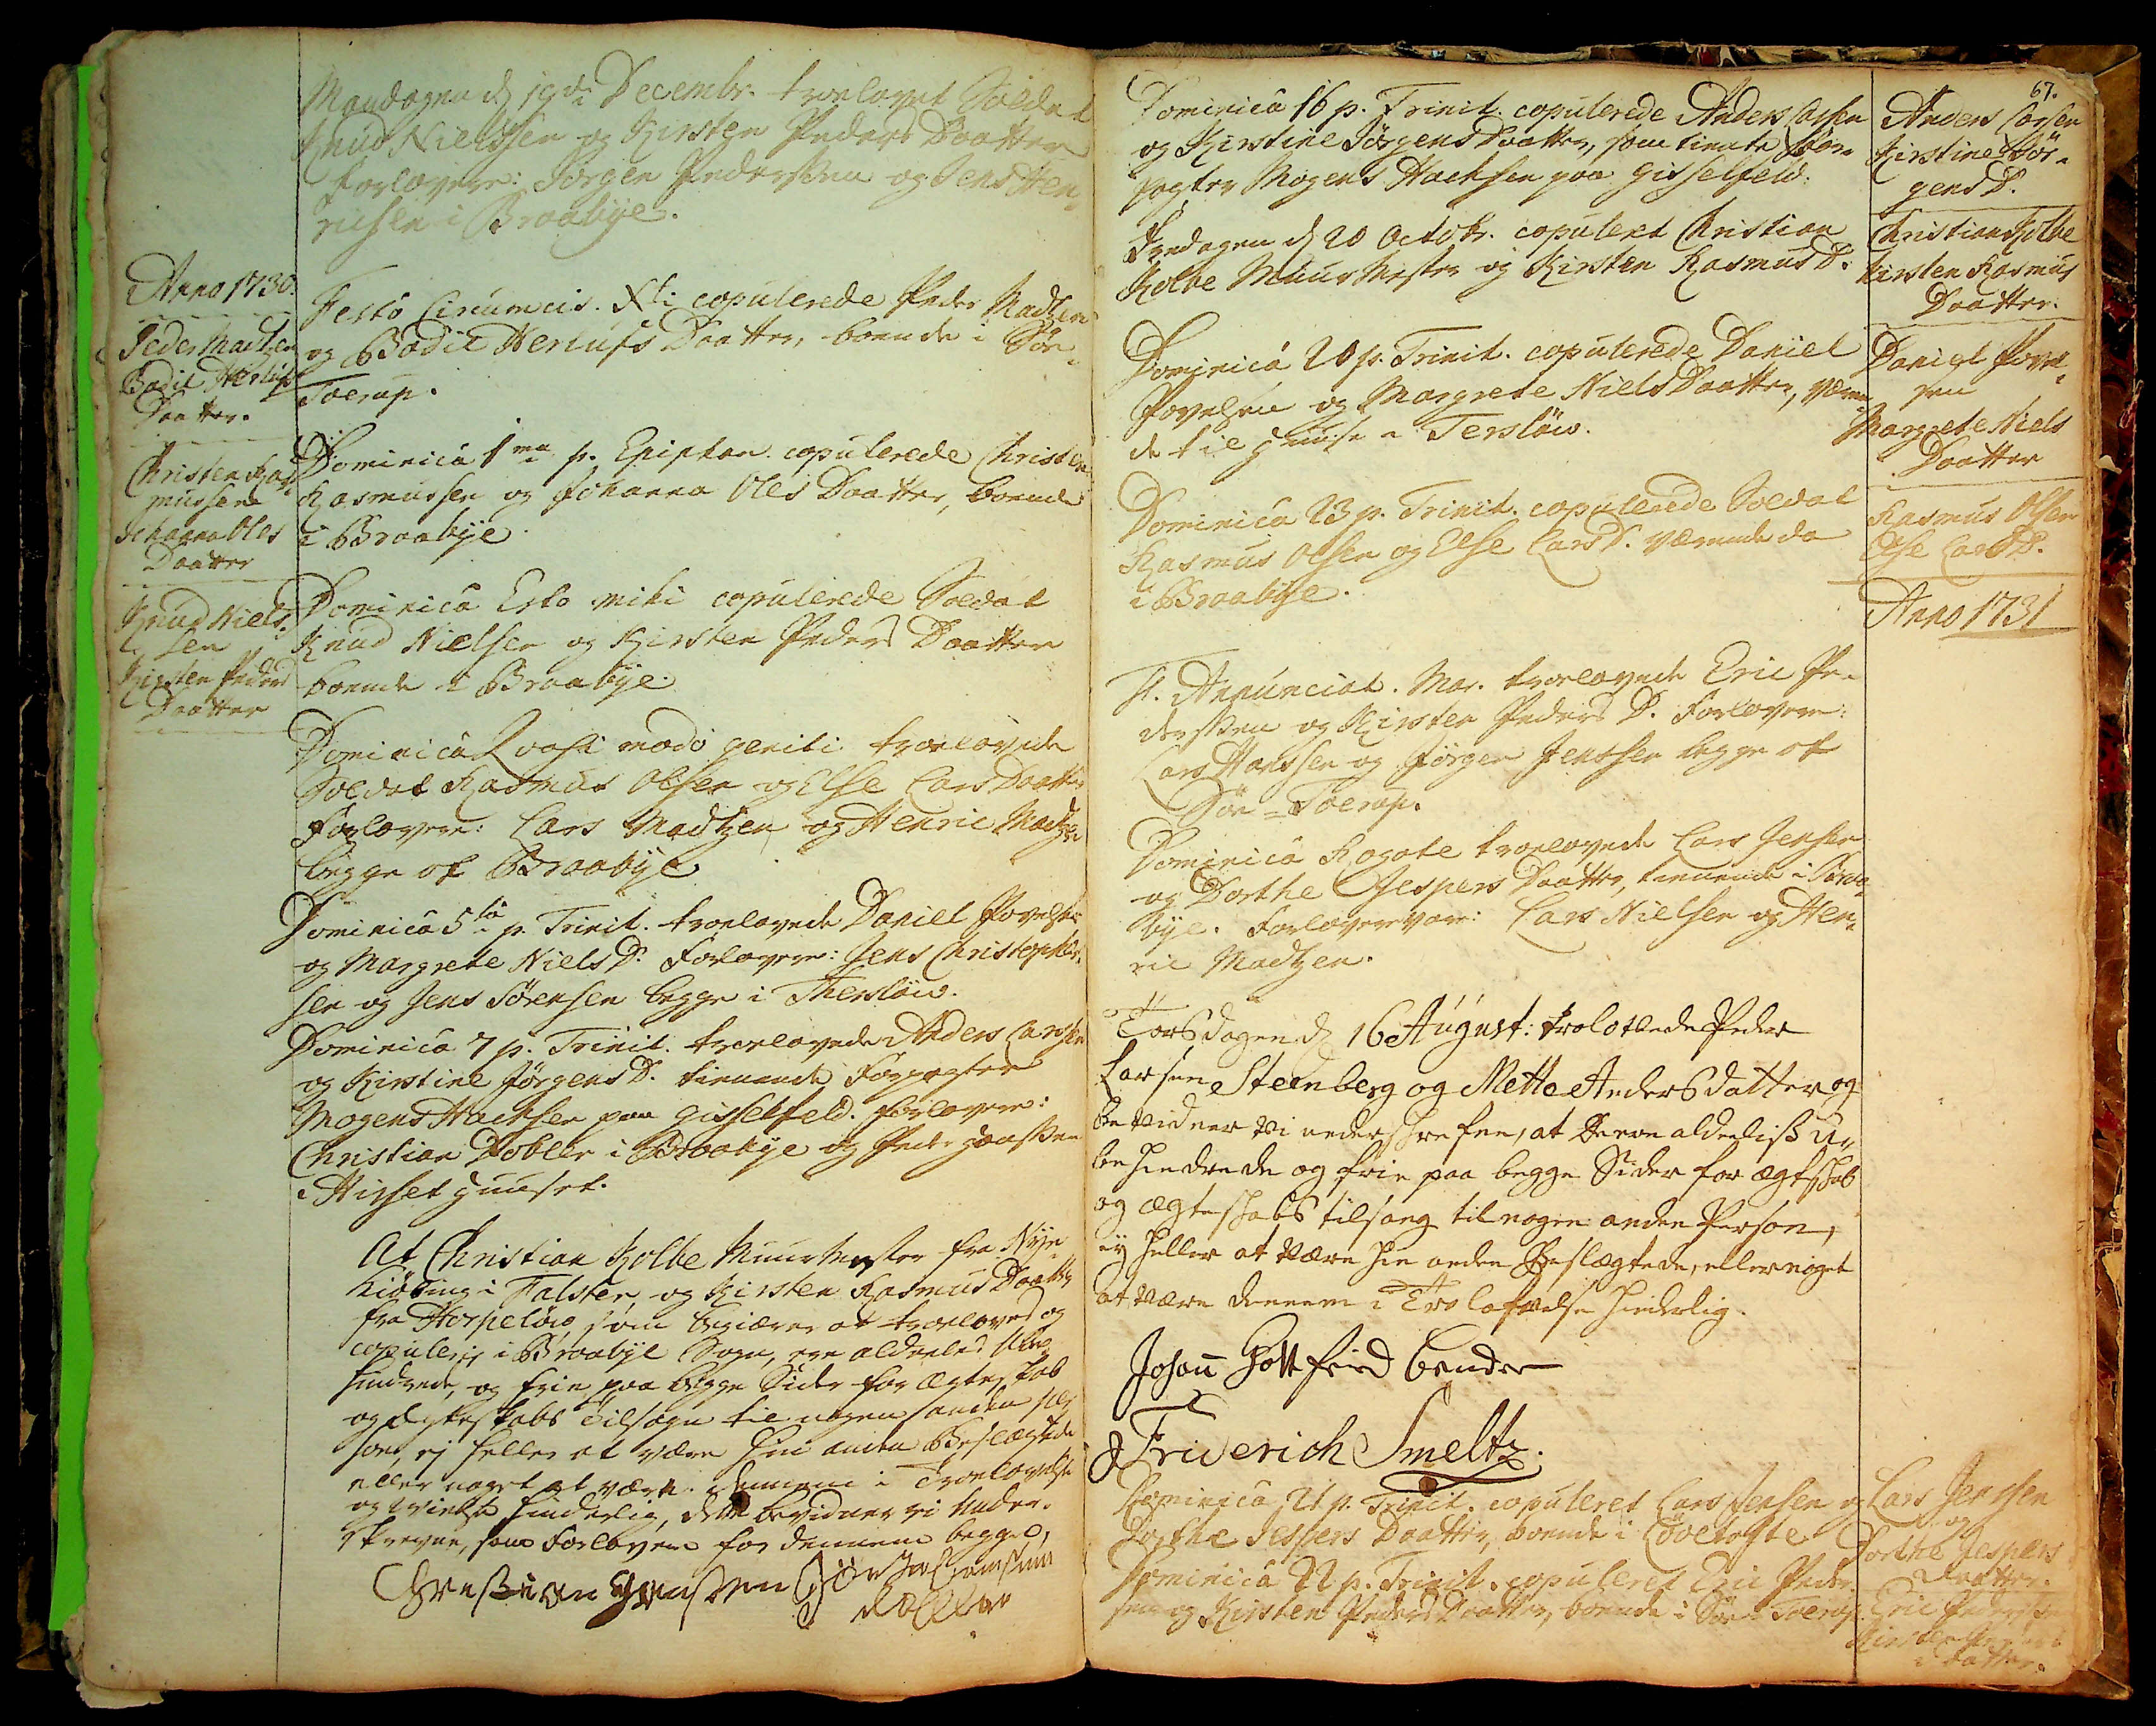Mandagen d. 19da## Decembr. troelovet Soldat
Knud Nielssen og Kirsten Peders Daatter.
Forlovere: Jørgen Pederssen og Jens Hen¬
ricsen i Braabye. 
Anno 1730
Peder Madtzen 
Bodil Herlufs
Daatter 
Festo Circumcis. Xte copulerede Peder Madtzen
og Bodil Herlufs Daatter, boende i Søe 
Toerup.
Christen Ras¬
mussen 
Johanna Oles
Daatter 
Dominica 1ma p. Epiphan. copulerede Christen
Rasmussen og Johanna Oles Daatter, boende
i Braabye.
Knud Niels¬
sen 
Kirsten Peders
Daatter
Dominica Esto Mihi copulerede Soldat
Knud Nielsen og Kirsten Peders Daatter
boende i Braabye. 
Dominica Quasimodo geniti troelovede
Soldat Rasmus Olsen og Else Lars Daatter.
Forlovere: Lars Madtzen og Henric Madtzen
begge af Braabye.
Dominica 5ta p. Trinit. troelovet Daniel Povelsen
og Margrete NielsD. Forlovere: Jens Christopher¬
sen og Jens Sørensen, begge i Frersløv.
Dominica 7 p. Trinit. troelovede Anders Larsen
og Kirstine JørgensD. tienende Forpagter
Mogens Hacksen## paa Gisselfeld. Forlovere:
Christian Dobler i Braabye og Peder Hanssen
i Hirset Huuset.
At Christian Kolbe MuurMester fra Nye¬
Kiøbing i Falster, og Kirsten Rasmus Daatter
fra Horpeløv, som begiærer at troeloves og
copuleris i Braabye Sogn, ere aldeeles uden
hindrer og frie paa begge sider for Ægteskab
og Ægteskabs Tilsagn til nogen anden per
son ej heller at være Hinanden Beslægtede
eller noget at være## dennem i Trolovelse
og Vielse hinderlig, dette bevidner vi under¬
skrevne som Forlovere for dennem begge.
Christian Iversen## Jørgen Hansen##
Dobler##
Dominica 16 p. Trinit. copulerede Anders Larsen
og Kirstine Jørgens Daatter, som tiente For
pagter Mogens Hacksen## paa Gisselfeld.
67.
Anders Larsen
Kirstine Jør¬
gensD.
Fredagen d. 20 Octobr. copuleret Christian
Kolbe MuurMester og Kirsten RasmusD.
Christian Kolbe
Kirsten Rasmus
Daatter 
Dominica 20 p.Trinit copulerede Daniel 
Povelsen og Margrete Niels Daatter, væren¬
de til Huuse i Tersløv.
Daniel Povel¬
sen 
Margrete Niels 
Daatter 
Dominica 23 p. Trinit copulerede Soldat 
Rasmus Olsen og Else LarsD. Værende da
i Braabye.
Rasmus Olesen 
Else LarsD.
F. Annunciat Mar. troelovet Eric Pe¬
derssen og Kirsten PedersD. Forlovere:
Lars Hanssen og Jørgen Jenssen begge af
Søe Toerup.
Anno 1731
Dominica Rogate troelovet Lars Jenssen
og Dorthe Jespers Daatter tienende i Braa##¬
bye. Forlovere vare: Lars Nielsen og Hen¬
ric Madtzen.
Torsdagen d. 16 August: troelovede Peder
Larsen Steenberg og Mette Andersdatter og
bevidner vi underskrefne at de ere aldelis u¬
den## hindrende og frie paa begge Sider for Ægteskab
og Ægteskabs tilsang til nogen anden Person,
ey heller at Være hinanden Beslægtede eller noget
at Være dennem i Trolofvelse hinderlig.
Johan Gottfred bonde
Frederich Smeltz 
Dominica 21 p. Trinit. copuleret Lars Jensen og
Dorthe Jespers Daatter, boende i Løvetofte.
Lars Jensen 
og
Dorthe Jespers
Daatter.
Dominica 22 p. Trinit. copuleret Eric Peder¬
sen og Kirsten Peders Daatter, boende i Søe-Toerup.
Eric Pedersen
Kirsten Peders
Datter.
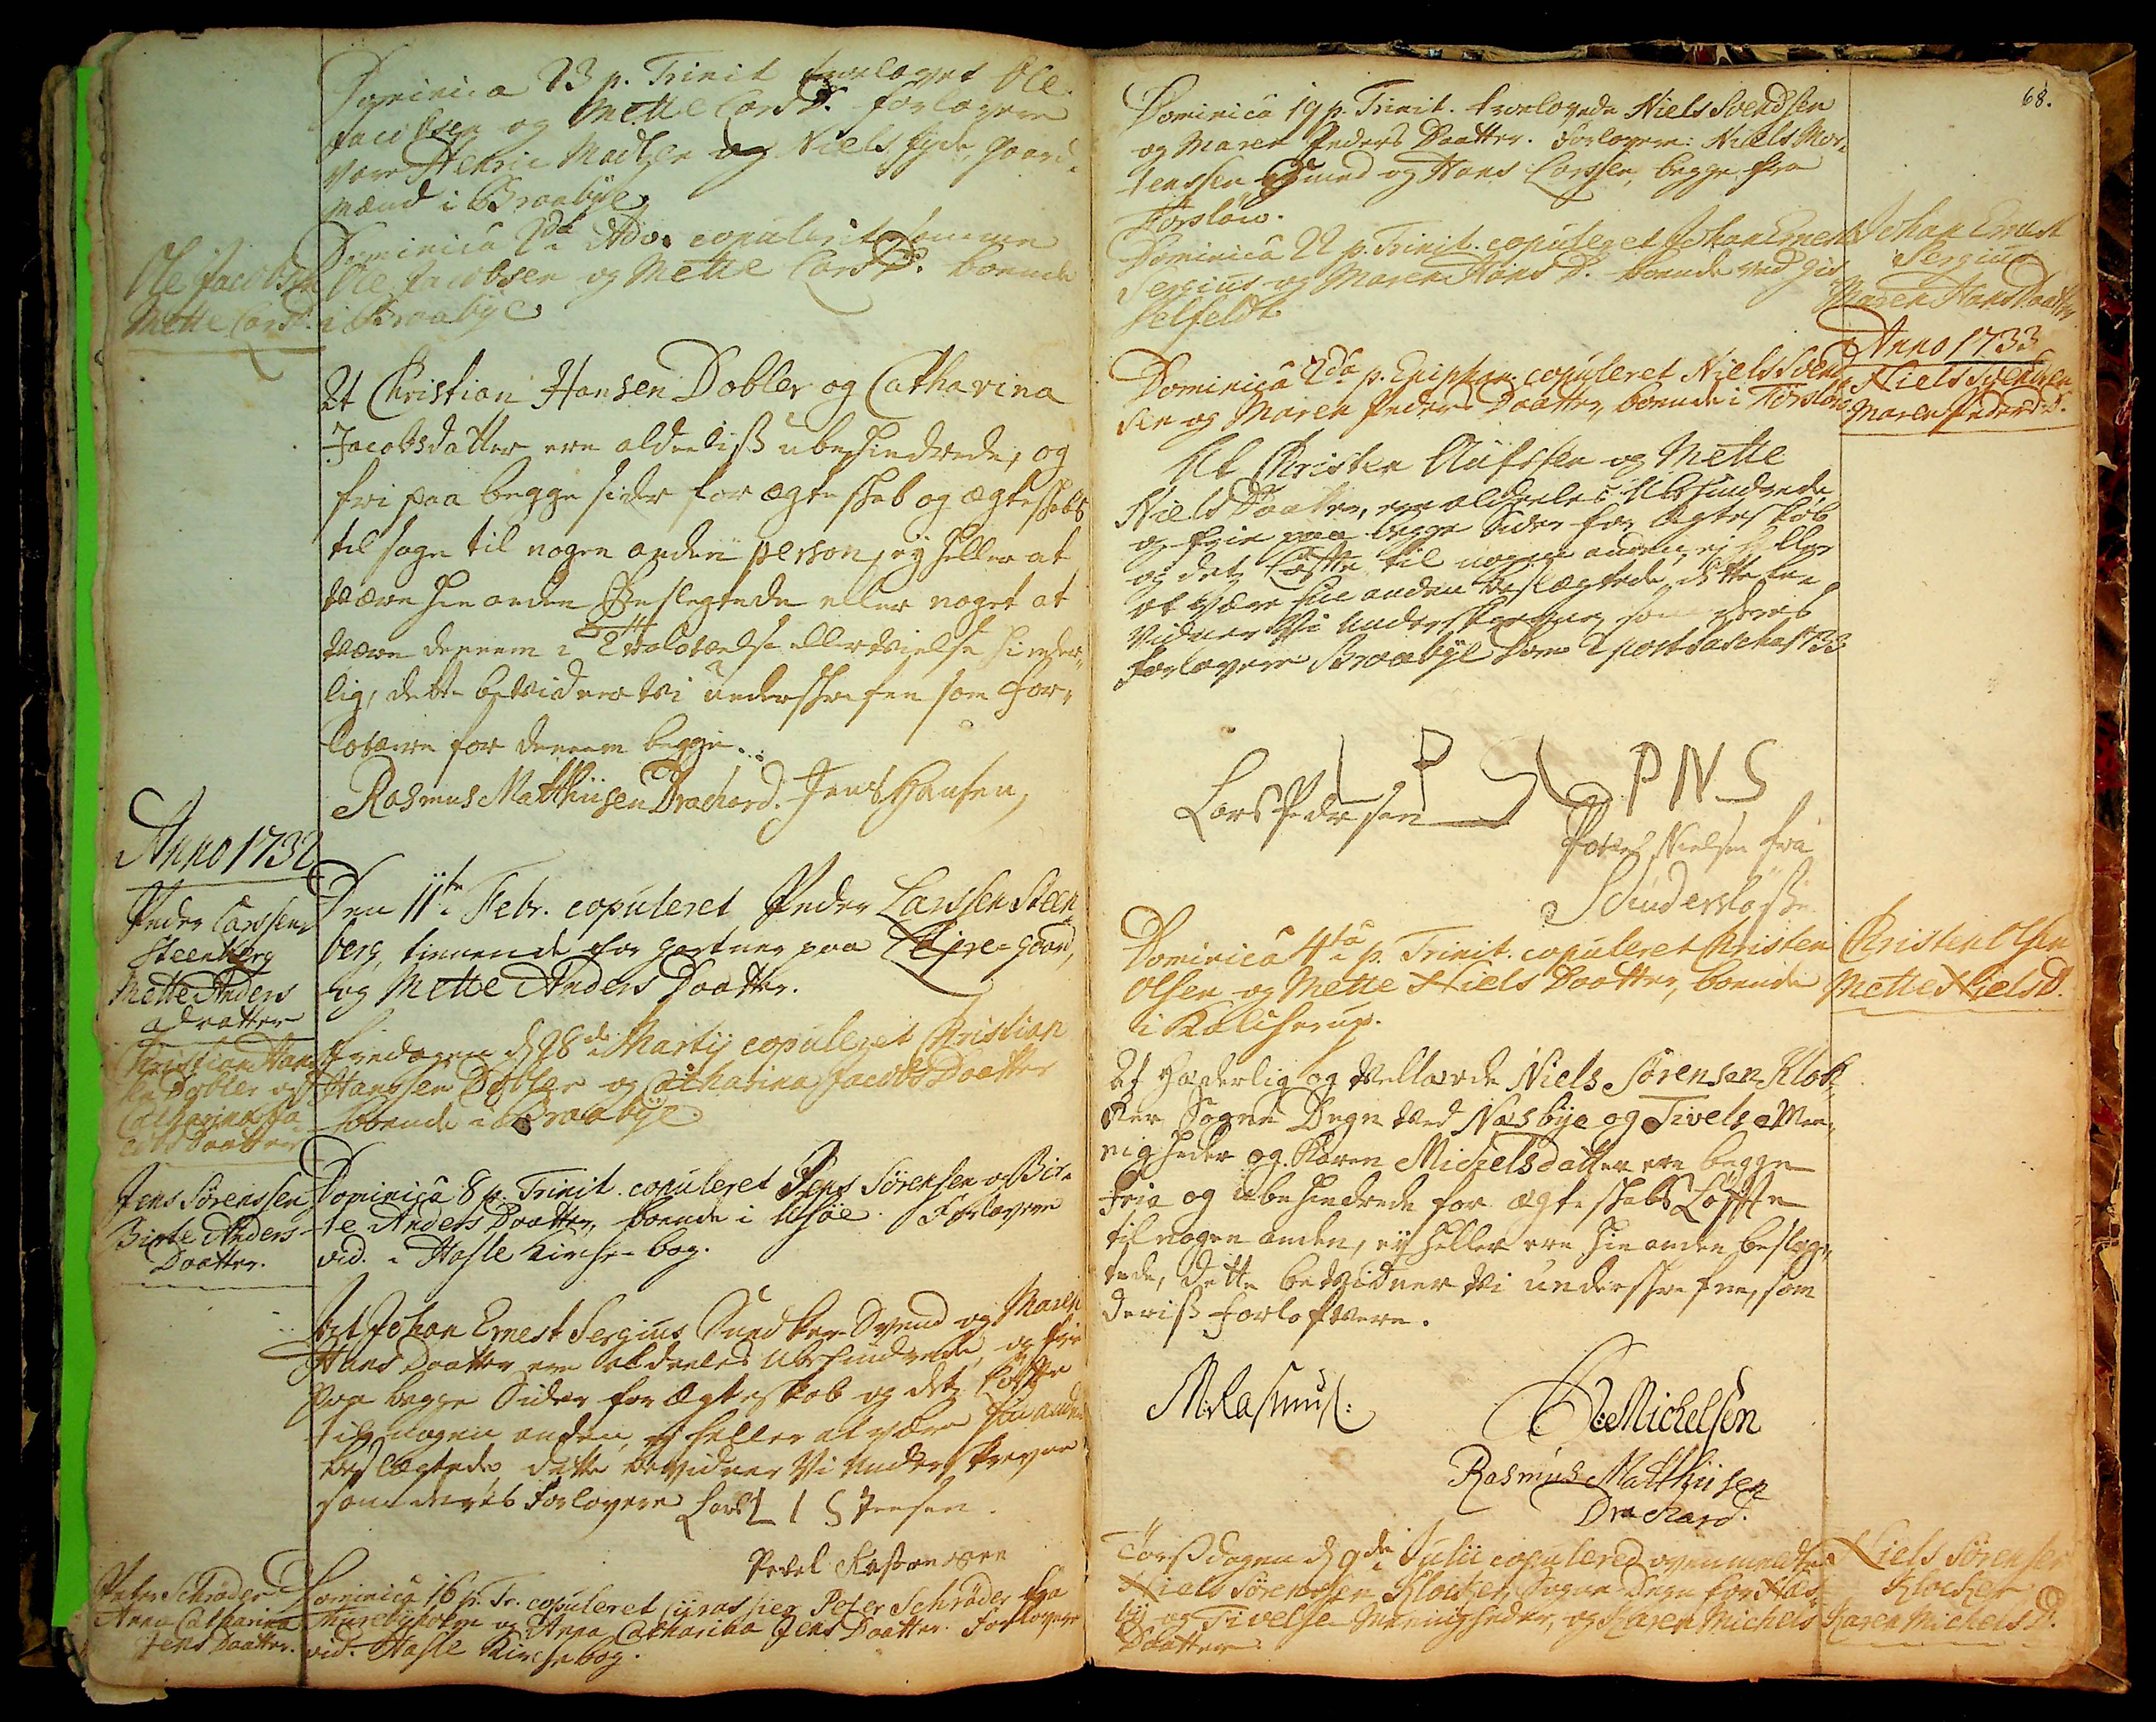Dominica 23 p. Trinit. troelovet Ole
Jacobsen og Mette LarsD. Forlovere
vare Henric Madtzen og Niels Jyde Gaard¬
mænd i Braaby.
Ole Jacobsen 
Mette LarsD. 
Dominica 2da Adv. copulerit samme
Ole Jacobsen og Mette LarsD. boende
i Braabye. 
At Christian Hansen Dobler og Catharina
Jacobsdatter ere aldeliss ubehindrede, og
fri paa begge Sider for Ægteskab og Ægteskabs
tilsagn til nogen anden person, ej heller at
være hinanden Beslegtede eller noget at
være dennem i Trolovelse eller Vielse hindre¬
lig, dette bevidner vi underskrefne som For¬
lovere for dennem begge.
Rasmus Matthiisen Drachard. Jens Hansen
Anno 1732
Peder Larsen 
Steenberg
Mette Anders 
Daatter 
Den 11te Febr. copuleret Peder Larssen Steen¬
berg, tienende for Gartner paa Lejre-gaard
og Mette Anders Daatter.
Christian Hans
sen## Dobler og
Catharina Ja
cobsDaatter 
Fredagen d. 28de Marty copulerit Christian
Hanssen Dobler og Catharina Jacobs Daatter,
boende i Braabye.
Jens Sørenssen
Birte Anders
Daatter. 
Dominica 8 p Trinit. copuleret Jens Sørensen og Bir¬
te Anders Daatter, boende i Ulsøe. Forlovere
vid. i Hasle Kirchebog.
At Johan Ernest Sergius Snedker Svend og Maren
Hans Daatter ere aldeeles ubehindrede og fri 
paa begge Sider for Ægteskab og dets Løffte 
til nogen anden ej heller at være hinanden
beslægtede dette bevidner Vi Underskrevne
som deres Forlovere Lars L I S Jensen
Povel Rasmussen##
Peter Schröder
Anna Catharina 
Jens Daatter
Dominica 16 p. Tr. copuleret Cyrassier Peter Schrøder fra
Thurebyholm## og Anna Catharina Jens Daatter. Forlovere
vid i Hasle Kirchebog.
Dominica 19 p. Trinit. troelovede Niels Svendsen
og Maren Peders Daatter. Forlovere: Niels Mor¬
tenssen Smed og Hans Larssen, begge fra
Førslev.
68.
Dominica 22 p, Trinit. copuleret Johan Ernest
Sergius og Maren HansD. boende ved Gis
selfeldt.
Johan Ernest
Sergius 
Maren HansDaatter 
Dominica 2da p.Epiphan. copuleret Niels Svend¬
sen og Maren Peders Daatter, boende i Førsløv.
Anno 1733 
Niels Svendsen 
Maren PedersD.
At Christen Olufsen og Mette
Niels Daatter ere aldeeles Ubehindrede
og frie paa begge sider for Ægteskab
og dets Løffte til nogen anden ej heller
at være hinanden beslægtede: dette be¬
vidner Vi underskrefne som deres
Forlovere Braabye Dom 2 post. Pachatos## 1733.
L P S
Lars Pedersen
P N S
Pofuel Nielsen fra
Skudersløse
Dominica 4ta p. Trinit. copuleret Christen
Olsen og Mette Niels Daatter, boende
i Kalcherup.
Christen Olsen 
Mette NielsD. 
At Hæderlig og Vellærde Niels Sørensen Klok¬
ker Sogne Degn ved Næsbye og Tivelse Me¬
enigheder og Karen Michelsdatter ere begge
frie og ubehindrede for Ægteskabs Løffte
til nogen anden, ej heller ere Hinanden beslæg¬
tede dette bedvidner Vi underskrefne som
deris Forlofvere.
M: Rasmuss:
S## Michelsen
Rasmus Matthiisen
Drachard
Torsdagen d. 9d July copulered ovenmeldte
Niels Sørensen Klocker, SogneDegn for Næs¬
by og Tivelse Menigheder, og Karen Michels
Daatter.
Niels Sørensen 
Klocker 
Karen MichelsD.